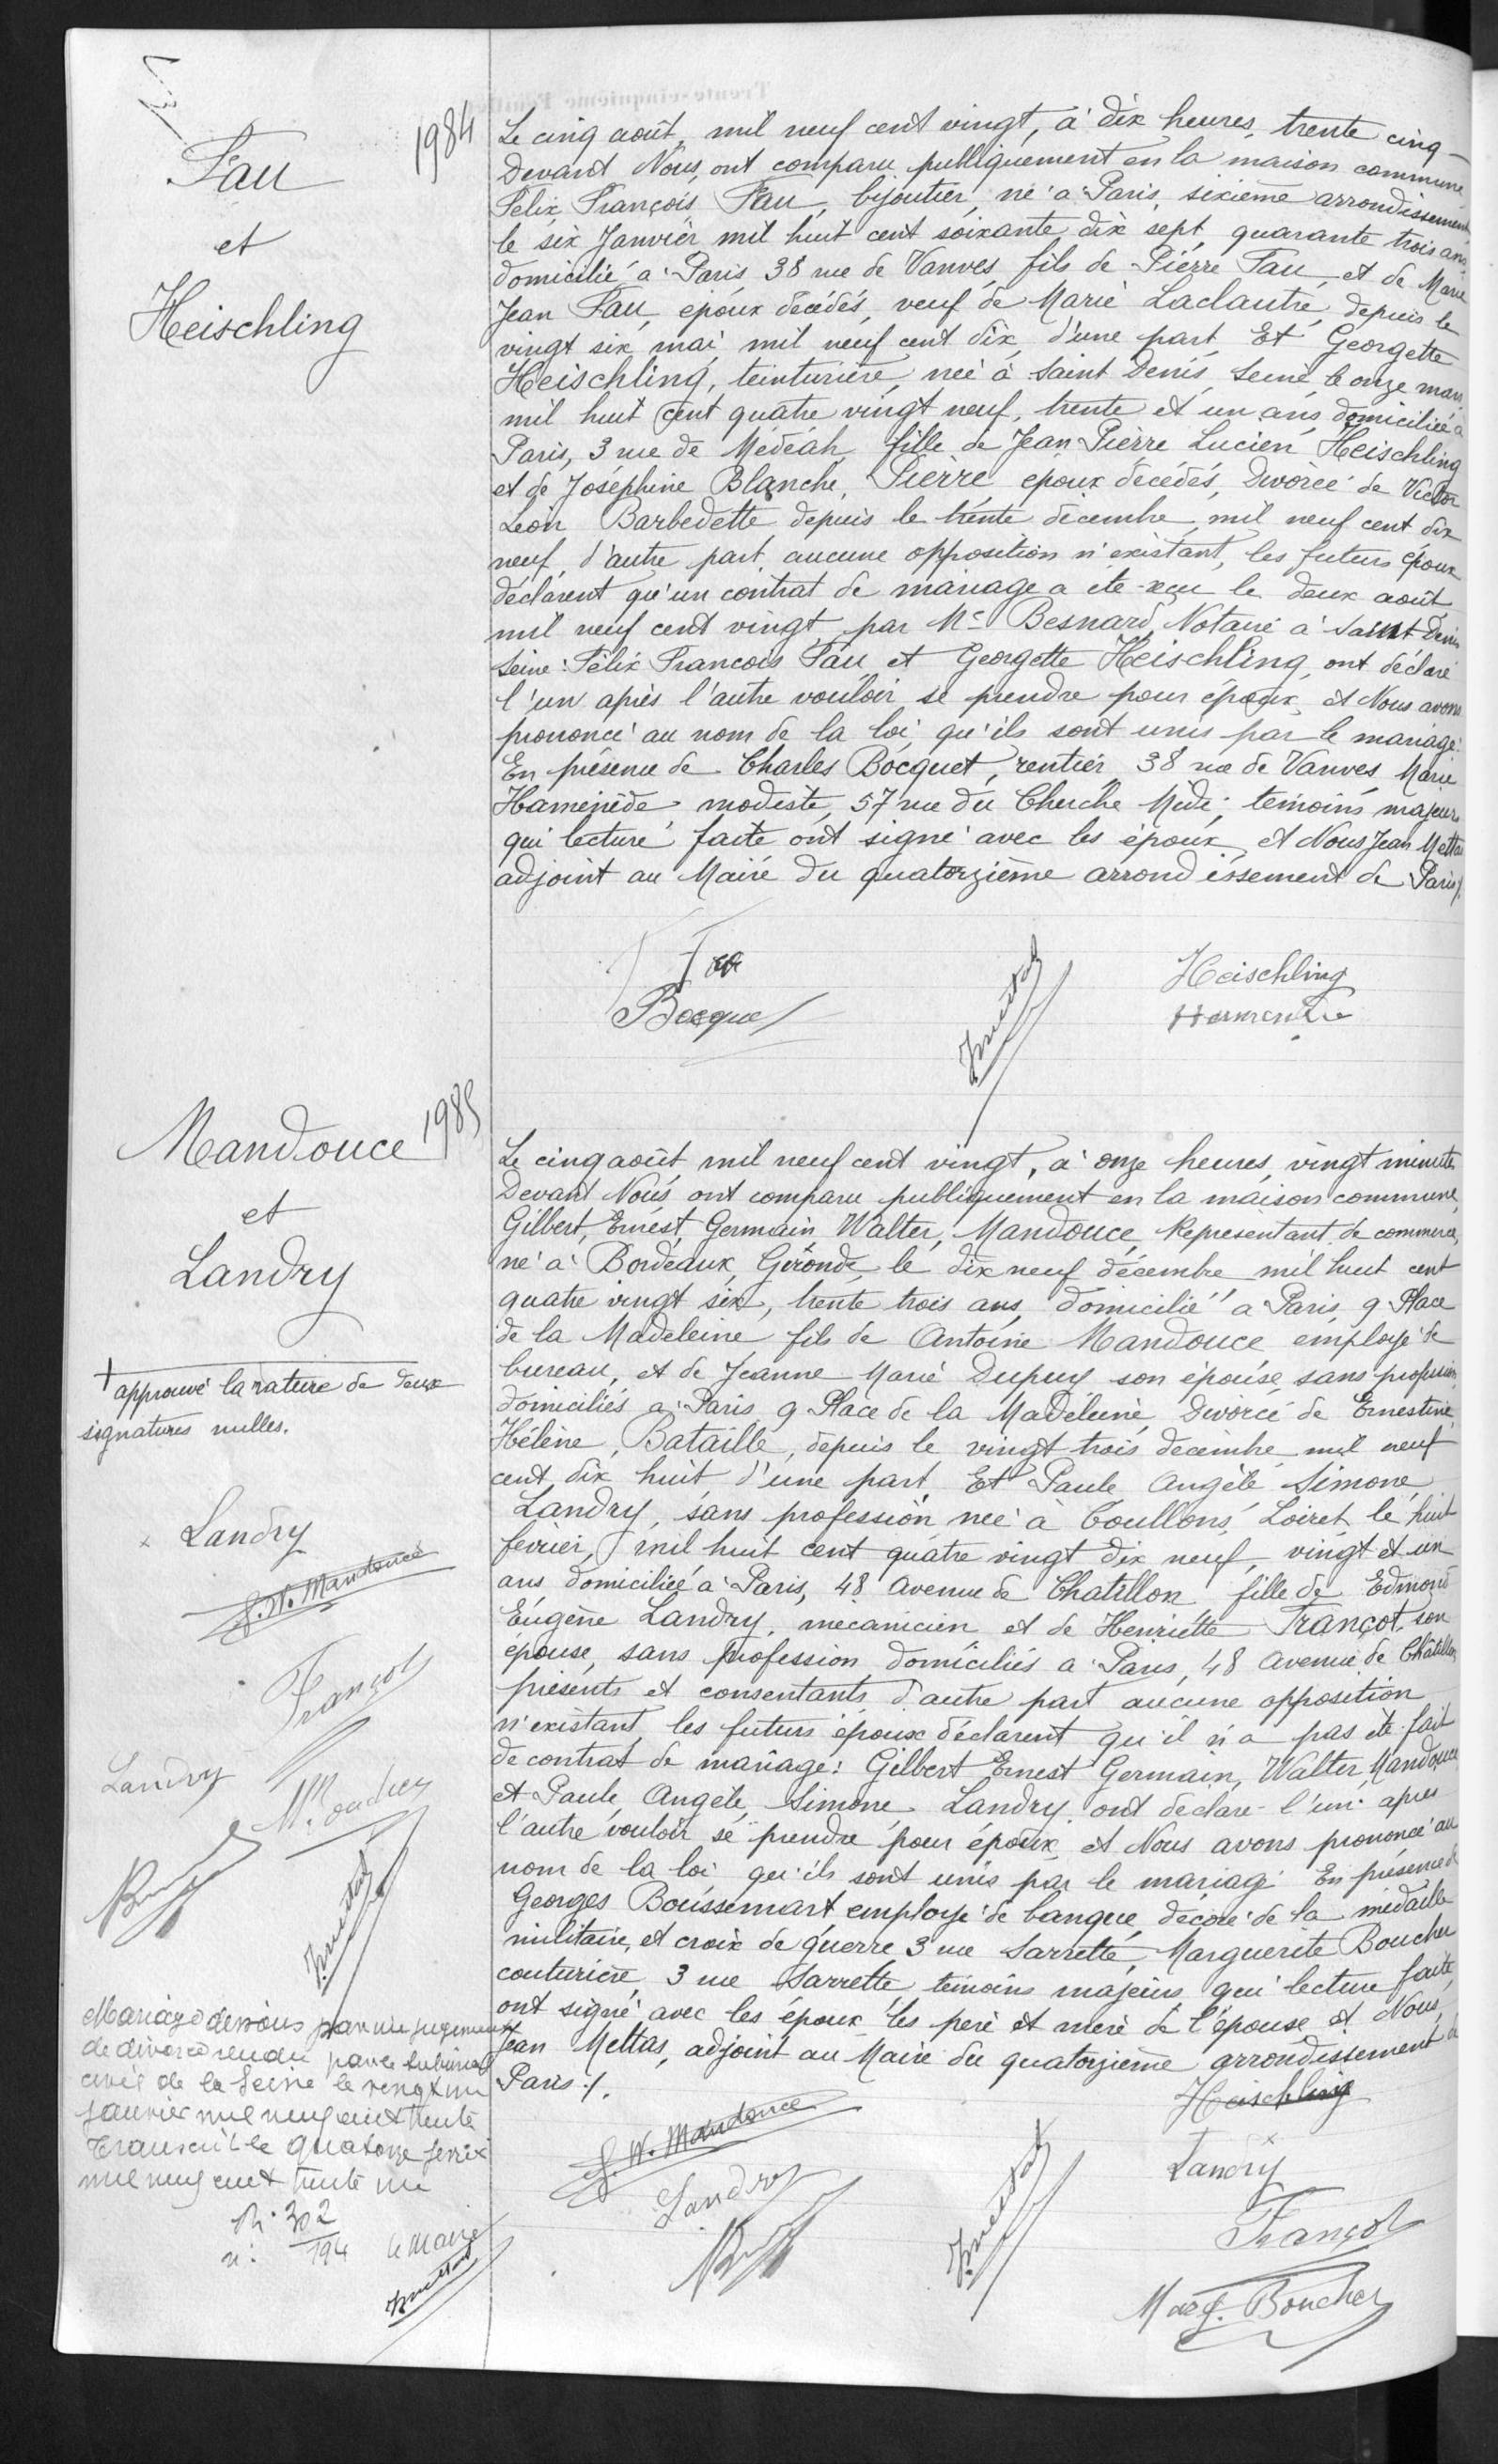Fau 1984
et
Heischeling
Le cinq août mil neuf cent vingt, à dix heures trente cinq
Devant Nous ont comparu publiquement en la maison commune
Félix François Fau, bijoutier, né à Paris, sixième arrondissement
le six Janvier mil huit cent soixante dix sept, quarante trois ans
domicilié à Paris 38 rue de Vanves fils de Pierre Fau, et de Marie
Jean Fau, époux décédés, veuf de Marie Laclautré, depuis le
vingt six mai mil neuf cent dix, d'une part, Et Georgette
Heischling, teinturière, née à Saint Denis, Seine, le onze mars
mil huit cent quatre vingt neuf, trente et un ans domiciliée à
Paris, 3 rue de Médéah fille de Jean Pierre Lucien Heischling
et de Joséphine Blanche Pierre époux décédés, divorcée de Victor
Léon Barbedette depuis le trente décembre mil neuf cent dix
neuf, d'autre part, aucune opposition n'existant, les futurs époux
déclarent qu'un contrat de mariage a été reçu le deux août
mil neuf cent vingt par Me Besnard Notaire à Saint Denis
Seine Félix François Fau et Georgette Heischling, ont déclaré
l'un après l'autre vouloir se prendre pour époux et Nous avons
prononcé au nom de la loi, qu'ils sont unis par le mariage.
En présence de Charles Bocquet, rentier 38 rue de Vanves, Marie
Haminède, modiste, 57 rue du Cherche Midi; témoins majeur
qui lecture faite ont signé avec les époux et Nous Jean Metta
adjoint au Maire du quatorzième arrondissement de Paris ./.
Mandouce 1985
et
Landry
Le cinq août mil neuf cent vingt, à onze heures, vingt minute
Devant Nous ont comparu publiquement en la maison commune,
Gilbert, Ernest, Germain, Walter, Mandouce, représentant de commerce
né à Bordeaux, Gironde le dix neuf décembre mil huit cent
quatre vingt six, trente trois ans, domicilié à Paris, 9 Place
de la Madeleine fils de Antoine Mandouce employé de
bureau, et de Jeanne Marie Duppuy son épouse sans profession
domicilié à Paris 9 Place de la Madeleine, Divorcé de Ernestine
Hélène, Bataille, depuis le vingt trois décembre mil neuf
cent di huit d'une part. Et Paule Angèle Simone
Landry, sans profession née à Coullons, Loiret le huit
février mil huit cent quatre vingt dix neuf, vingt et un
ans, domiciliée à Paris, 48 Avenue de Chatillon fille de Edmond
Eugène Landry, mécanicien et de Henriette Francot son
épouse, sans profession domiciliés à Paris, 48 avenue de Châtillon
présents et consentants d'autre part aucune opposition
n'existant les futurs époux déclarent qu'il n'a pas été fait
de contrat de mariage: Gilbert Ernest Germain, Walter Mandouce
et Paule, Angèle, Simone Landry ont déclaré l'un après
l'autre vouloir se prendre pour époux et Nous avons prononcé au
nom de la loi qu'ils sont unis par le mariage. En présence de
Georges Boussemart employé de banque, décoré de la médaille
militaire et croix de guerre 3 rue Sarrette, Marguerite Bouchu
couturière, 3 rue Sarrette témoins majeurs qui lecture faite,
ont signé avec les époux les père et mère de l'épouse et Nous
Jean Mettas, adjoint au Maire du quatorzième arrondissement de
Paris./.
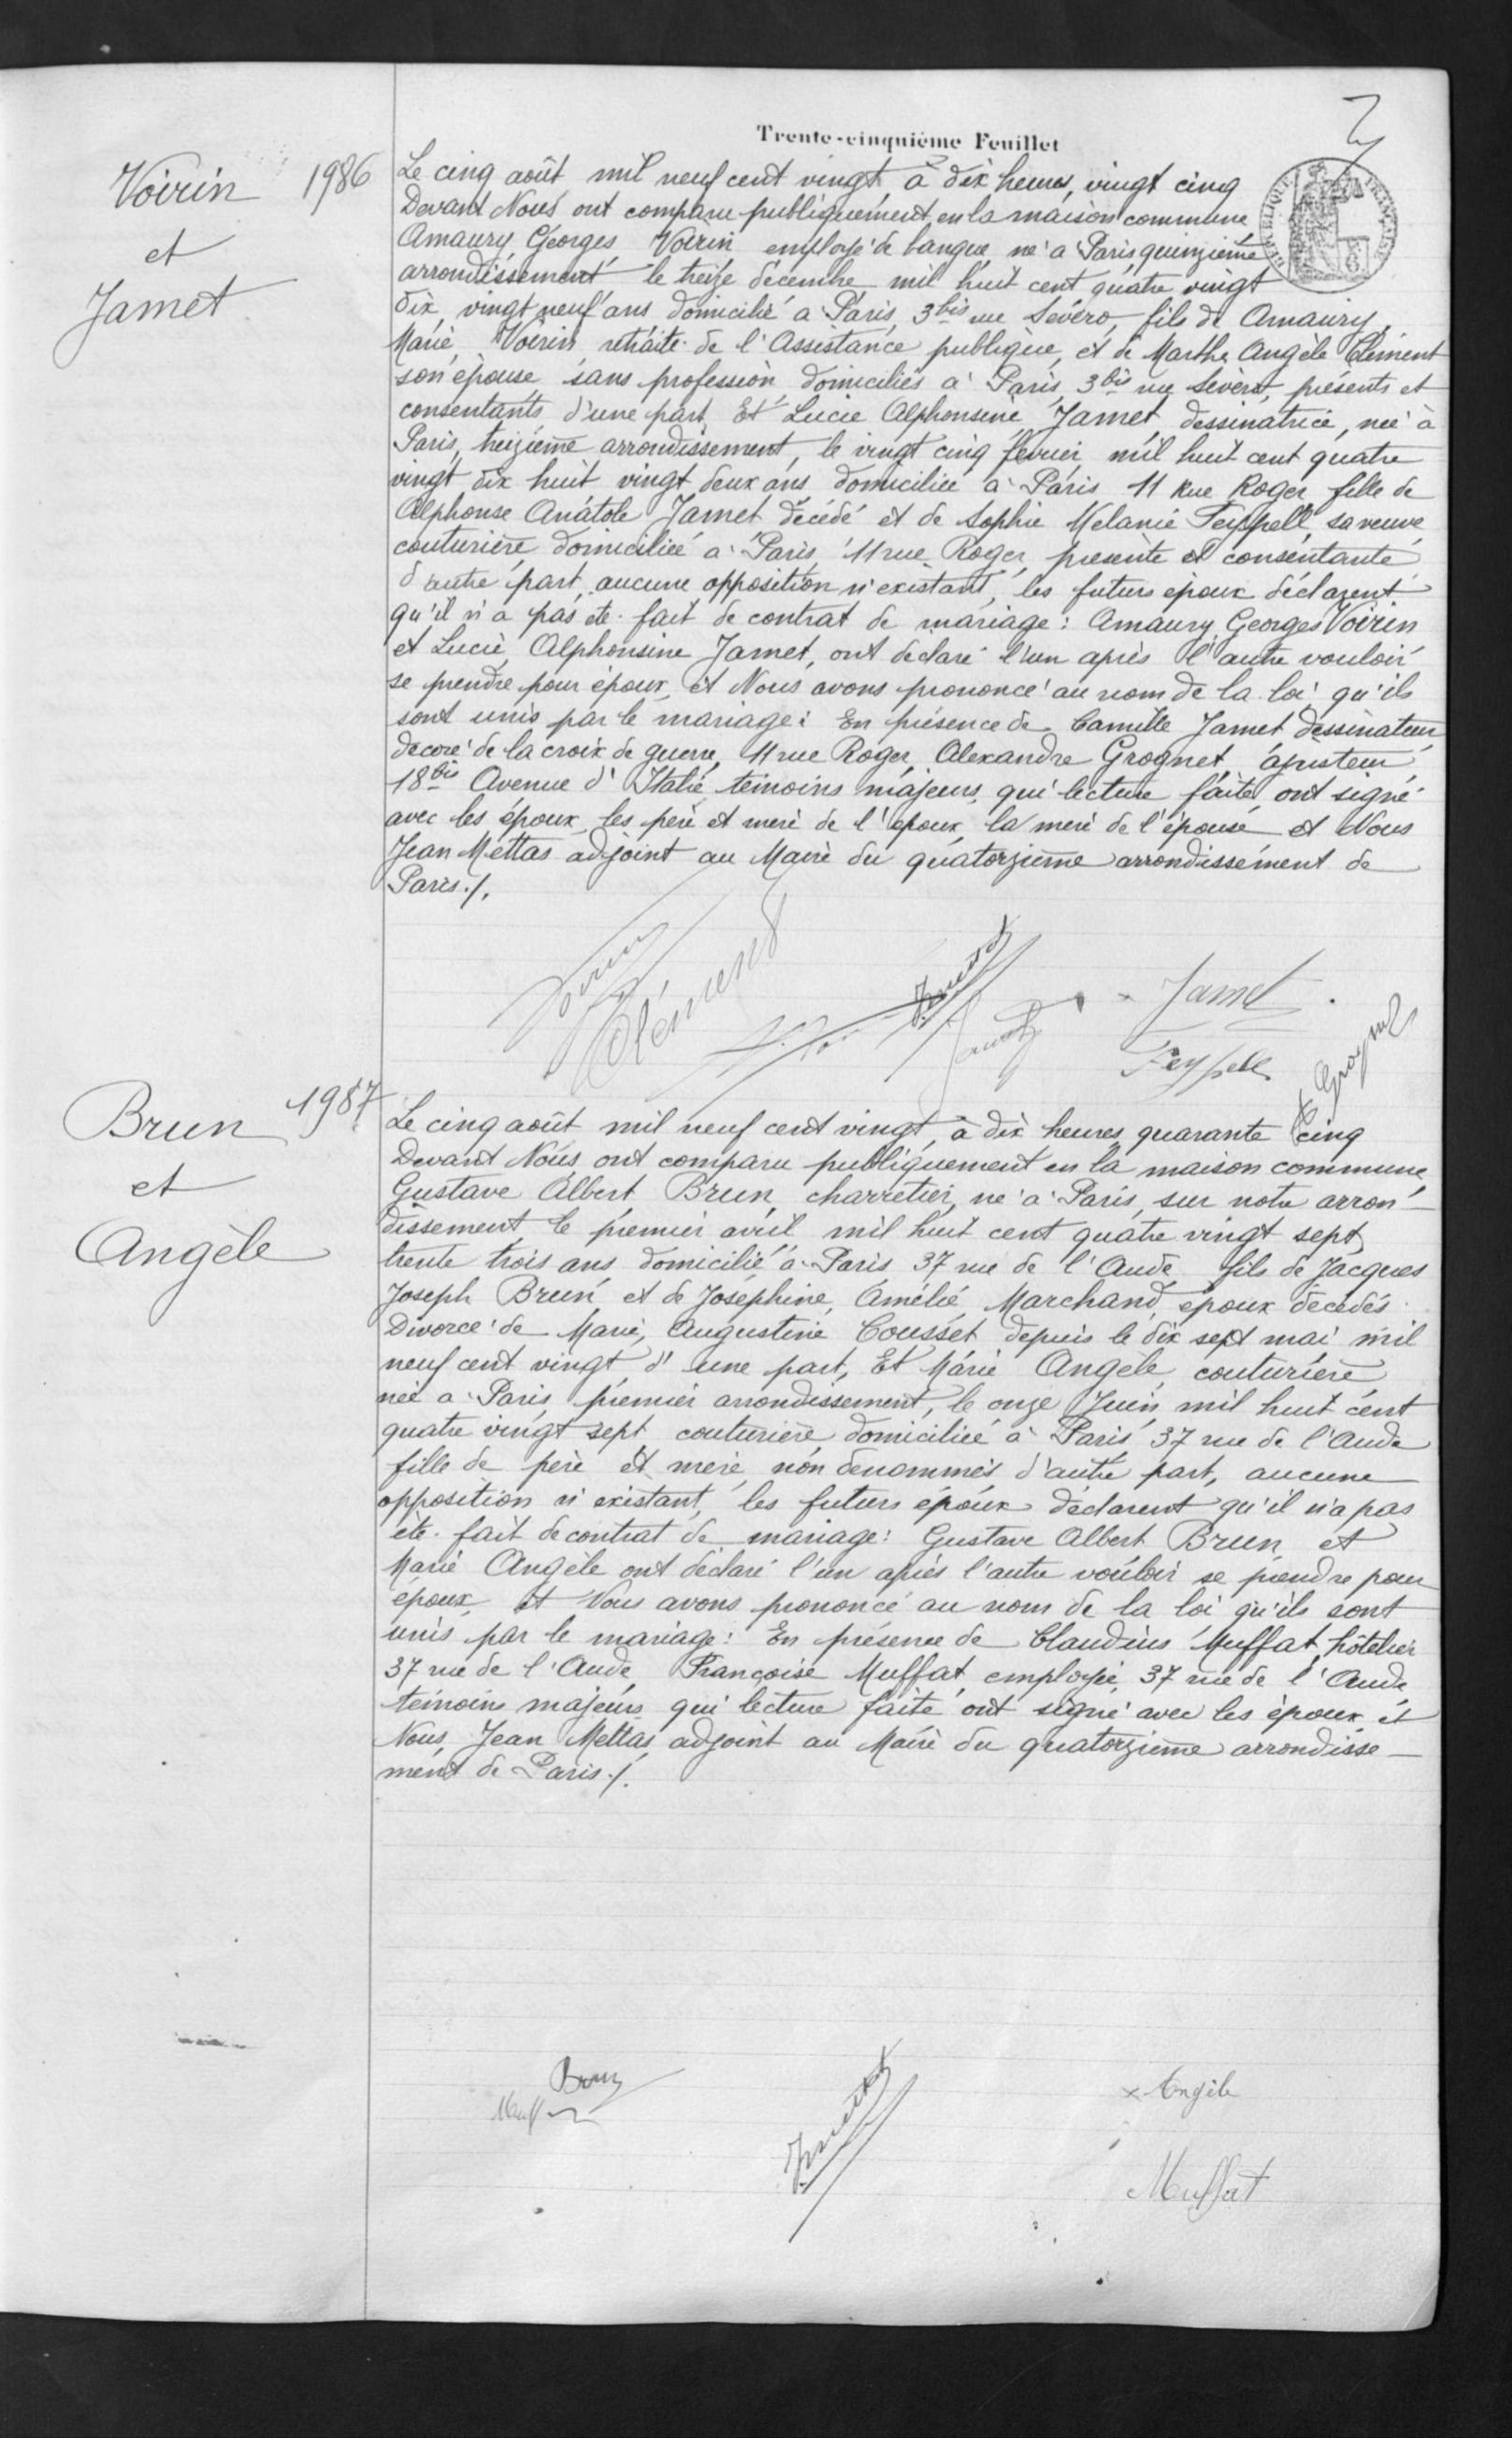Voirin 1986
et
Jamet
Le cinq août mil neuf cent vingt, à dix heures, vingt cinq
Devant Nous ont comparu publiquement en la maison commune
Amaury Georges Voirin employé de banque, né à Paris, quinzième
arrondissement le treize décembre mil huit cent quatre vingt
dix, vingt neuf ans domicilié à Paris 3bis rue Sévero, fils de Amaury
Marie, Voirin, retraité de l'Assistance publique, et de Marthe Angèle Clément
son épouse sans profession domiciliés à Paris, 3 bis rue Sevèrs, présents et
consentants d'une part, Et Lucie Alphonsine Jamet, dessinatrice, née à
Paris, treizième arrondissement, le vingt cinq février, mil huit cent quatre
vingt dix huit vingt deux ans domiciliée à Paris 11 Rue Roger, fille de
Alphonse Anatole Jarnet décédé et de Sophie Mélanie Peypell, sa veuve
couturière domiciliée à Paris, 11 rue Roger présente et consentante
d'autre part, aucune opposition n'existant, les futurs époux déclarent
qu'il n'a pas été fait de contrat de mariage: Amaury Georges Voirin,
et Lucie Alphonsine Jarnet, ont déclaré l'un après l'autre vouloir
se prendre pour époux, et Nous avons prononcé au nom de la loi qu'ils
sont unis par le mariage: En présence de Camille Jarnet dessinateur
décoré de la croix de guerre, 11 rue Roger, Alexandre Grognet, ajusteur
18bis Avenue d'Italie témoins majeurs qui lecture faite, ont signé
avec les époux les père et mère de l'époux, la mère de l'épouse et Nous
Jean Mettas adjoint au Maire du quatorzième arrondissement de
Paris./.
Brun 1987
et
Angèle
Le cinq août mil neuf cent vingt, à dix heures quarante cinq
Devant Nous ont comparu publiquement en la maison commune,
Gustave Albert Brun, charretier né à Paris, sur notre arron-
dissement le premier avril mil huit cent quatre vingt sept,
trente trois ans domicilié à Paris 37 rue de l'Aude fils de Jacques
Joseph Brun et de Joséphine, Amélie Marchand époux décédés.
Divorcé de Marie Augustine Cousset depuis le dix sept mai mil
neuf cent vingt d'une part, Et Marie Angèle couturière
née à Paris premier arrondissement, le onze Juin mil huit cent
quatre vingt sept couturière domiciliée à Paris 37 rue de l'Aude
fille de père et mère non denommés d'autre part, aucune
opposition n'existant les futurs époux déclarent qu'il n'a pas
été fait de contrat de mariage: Gustave Albert Brun, et
Marie Angèle ont déclaré l'un après l'autre vouloir se prendre pour
époux et Nous avons prononcé au nom de la loi qu'ils sont
unis par le mariage: En présence de Claudius Muffat hôtelier
37 rue de l'aude, Françoise Muffat employée 37 rue de l'Aude
témoins majeurs qui lecture faite ont signé avec les époux et
Nous Jean Mettas adjoint au Maire du quatorzième arrondisse-
ment de Paris ./.